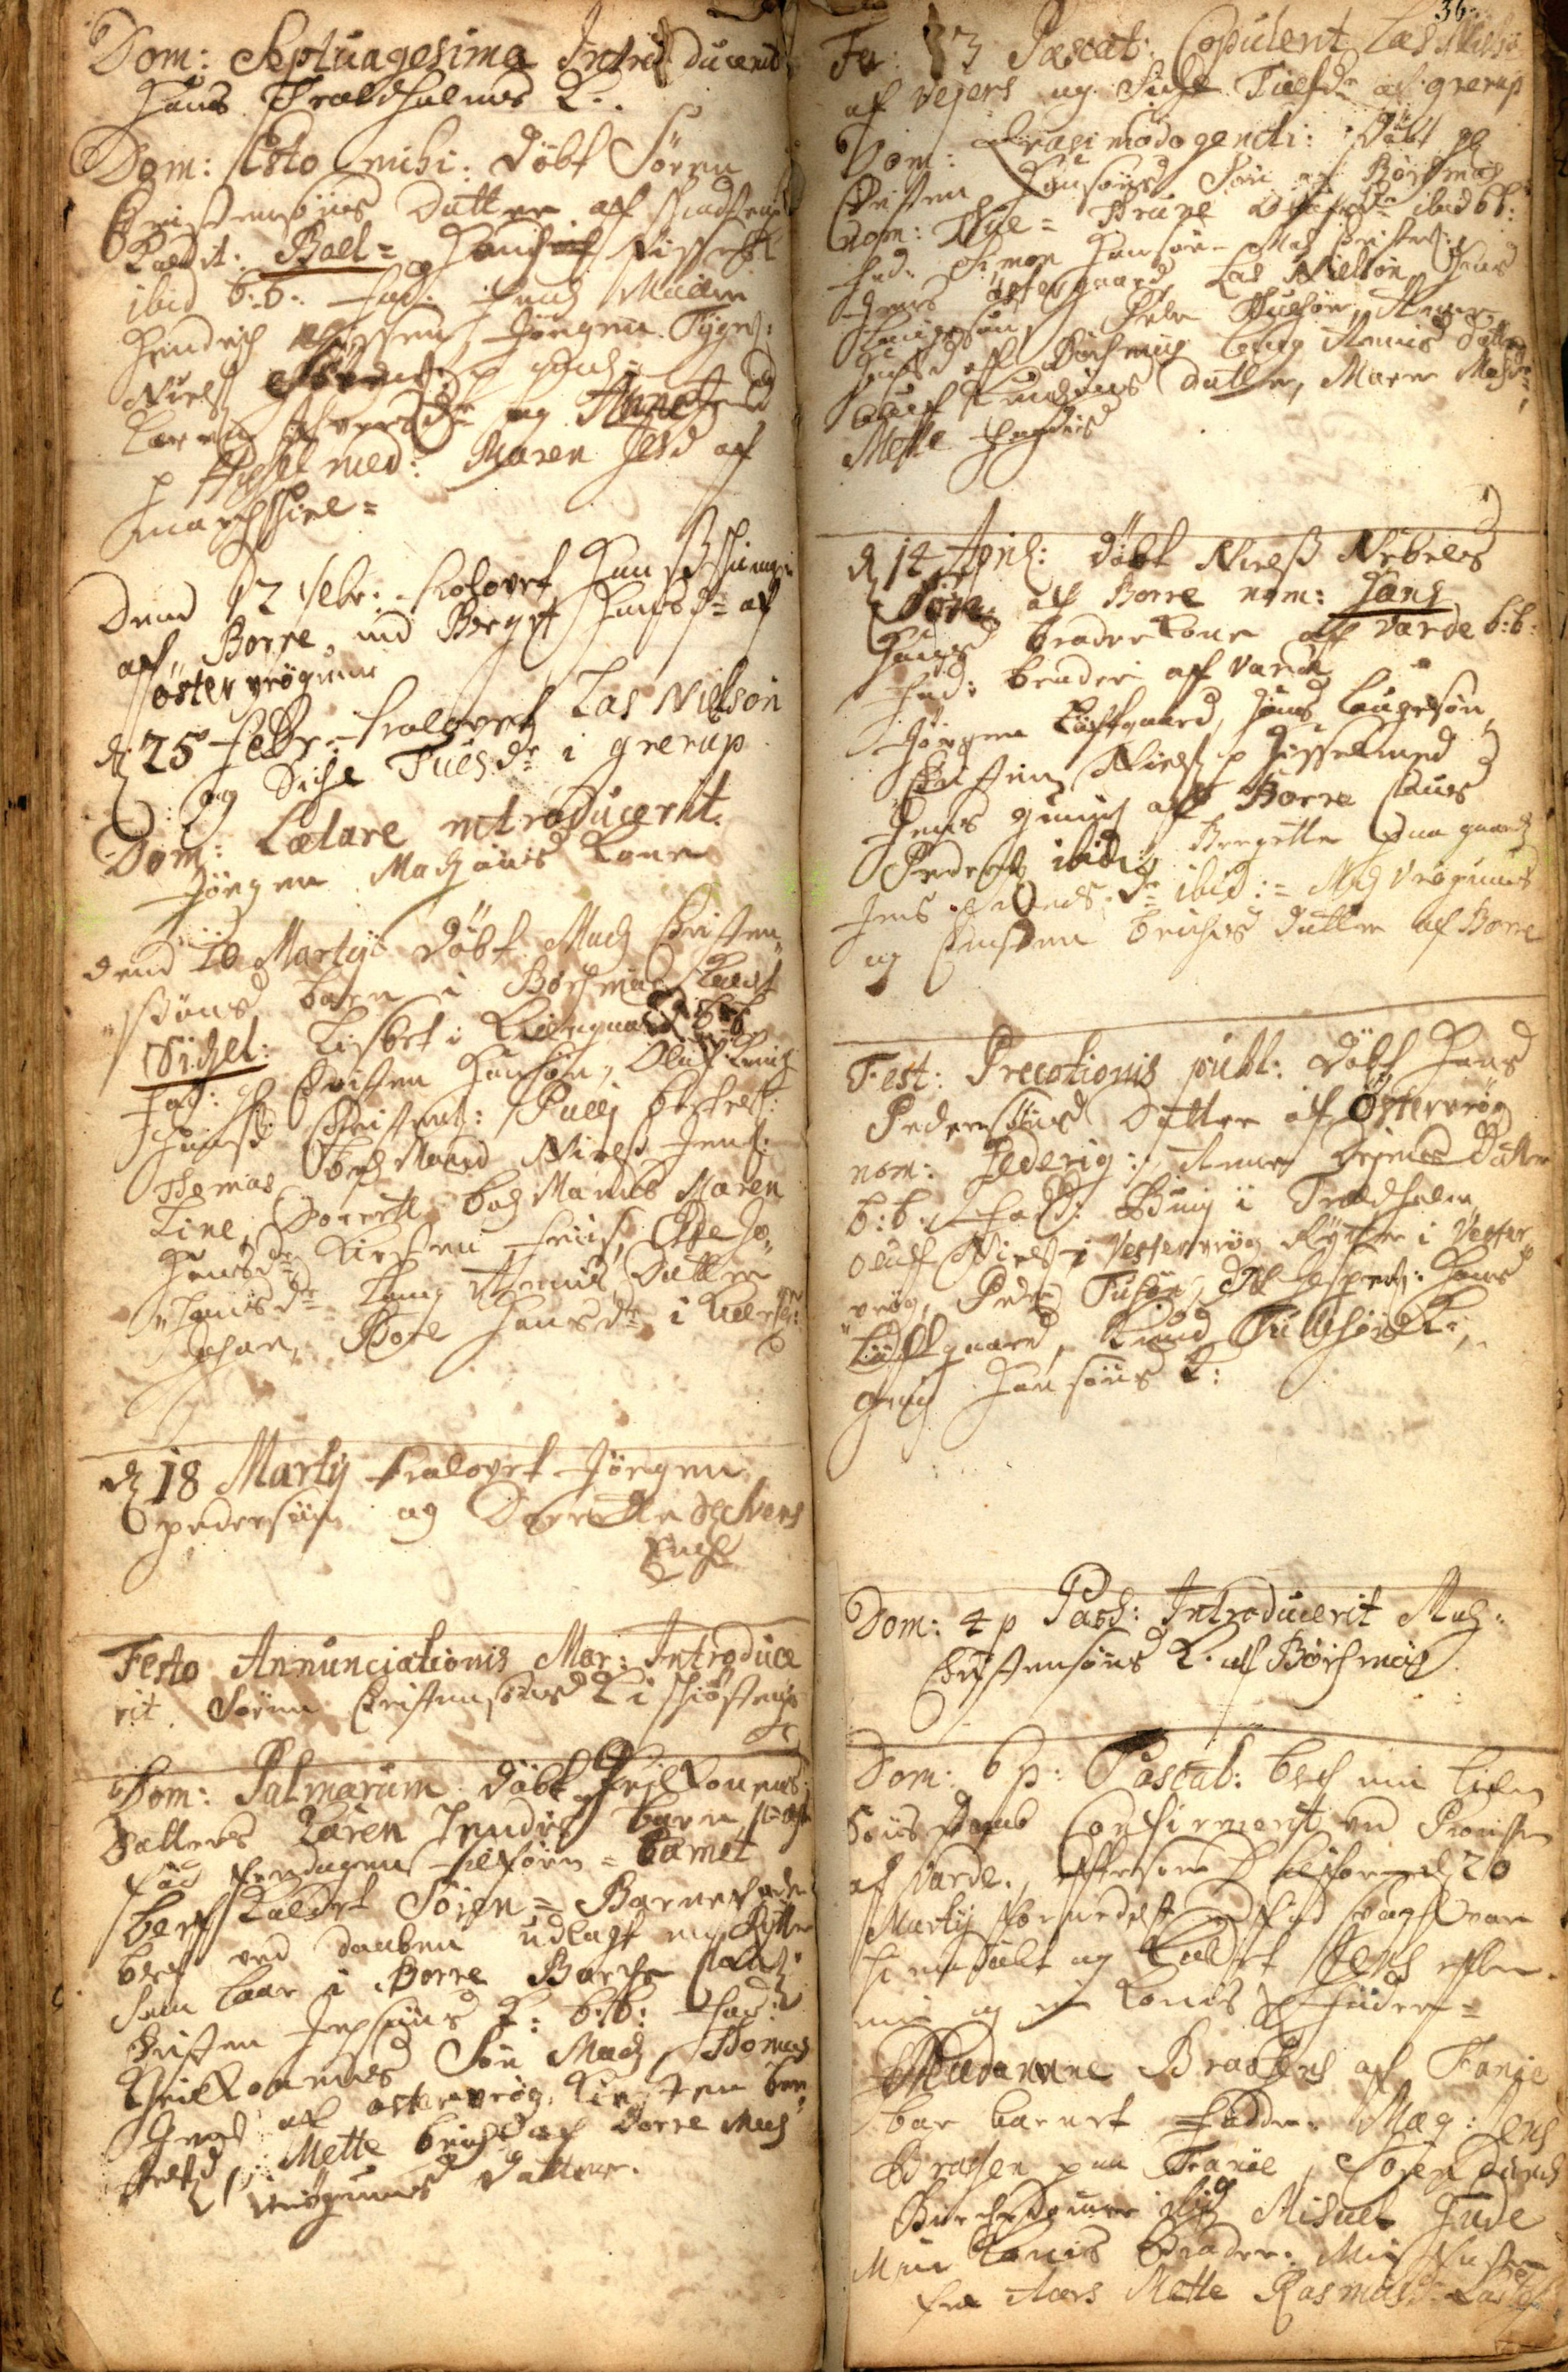DoM: Septuagesima Introducerit
Hans Troldholms K..
Dom: Esto Mihi: Døbt Søren
Christensøns Datter af Schiødstrup
kaldit: Boel= Hans ## Nisses K
ibid b:b: Fad: Frandz Møller
Hendrich Nissen, Jørgen Tyges:
Niels Sørens p ##=
Kirsten Ifversdr og Anne Jensd##
p Hisselmed: Maren Jesd af
Marchschiel=
Dend 12 Febr: trolovet Hans Schumager
af Borre med Bergit Hansdr af
Østervrøgum
D 25- Febr:- trolovet Las Nielsøn
og Siche## Tuesdr i Grerup.
Dom: Lætare introducerit
Jørgen Madzsøns Kone
Dend 10 Martij Døbt Madz Christen¬
søns Barn i Børsmøs kaldet
Sidzel: Lisbet iKiergaard b.b.
Fad: gl Christen Hansøn, Oluf Brich
Hans Christens: Pallj Bertels:
Thomas Bodz Mand Niels Jens:
Line, Dorrete Bodz Mands Maren
Hansdr Kiesten Friis, Else Jo¬
hansdr La## ##mis Datter
Johan, Boel Hansdr i Kiærgd##:
D 18 Martij trolovet Jørgen
Pedersøn og Dorrette Sl Ivers
Enche
Festo Annunciationis Mar.: Introduce
rit Søren Christensøns K i Schiødstrup
Dom: Palmarum Døbt Jejlkonens##
Datters Karen hendis Barn, ##
fød Fredagen tilforn= Barnet
blef kaldet Søren= Barnefader
blef ved Daaben udlagt en Rytter,
som laae i Borre Barchs Claus
Christen Jepsøns K: b:b: Fad:
Jeil##ouens Søn Madz, Thomas
G## af Østervrøg. Kirsten Ber¬
telsd,; Mette Brich af Borre Madz
Vrøgums Datter.
36
Fer: ## 3 Pascat: Copulerit Las Nielsøn
af Vejers og Sidzel Tuesdr af Grerup
Dom: Qvasimodogenit:i Døbt gl
Christen Hansøns Søn af Børsmos
nom: Thue= Thrine Olufsdr ibid bb:
Fad: Simon Hansøn, Madz Christens:
Jens Østergaard, Las Nielsøn, Hans
Laugesøn, Peder Thuesøn, Anne
Hansd af Børsmus ##g A##is Datter,
Oluf Knudzøns Datter, Maren Madzdr,
Mette Fogdies
D 14 April: Døbt Niels Nebels
Søn af Borre nom: Hans
Hans Broder Kone af Varde b:b:
Fad: Broder af Varde,
Jørgen Løftgaard, Hans Laugesøn,
Christen Niels p Hisselmed,
Jens Gunnis af Borre, Claus
Peders ibid: Bergitte paa gaard##
Jens Smeds## dr ibid:= Madz Vrøgums
og Christen Briches Datter af Borre
Fest: Precotionis publ: Døbt Hans
Pedersøns Datter af Østervrøg
nom: Hedevig: Anne Dejnens Datter
b:b: Fadd: Bunj i Troldholm
Oluf Niels i Vestervrøg Rytter i Vester¬
vrøg, Peder Tusøn, N Jespers: Hans
Løftgaard, Knud Tullesøn
G## Hansøns K:
Dom: 4 p Pasch: Introducerit Madz:
Christensøns K. af Børsmøs
Dom:6 p Pascat: Blef min liden
Søns Daab Confirmerit ved Prousten
af Varde. eftersom d tilforn d 20
Martij formedelst medfød Svaghed var
hiemmedøbt og kaldet Jens efter
min og min Konis Sl Fader=
Madamme Braachens af Fanøe
bar Barnet. Faddere Mag. Jens
Brachen paa Fanøe, Søren Du##
B##chedemus ibid, Michael Gude
Min Konis Broder: Min Søster
fra Aars## Mette Rasmusdr Lassøn
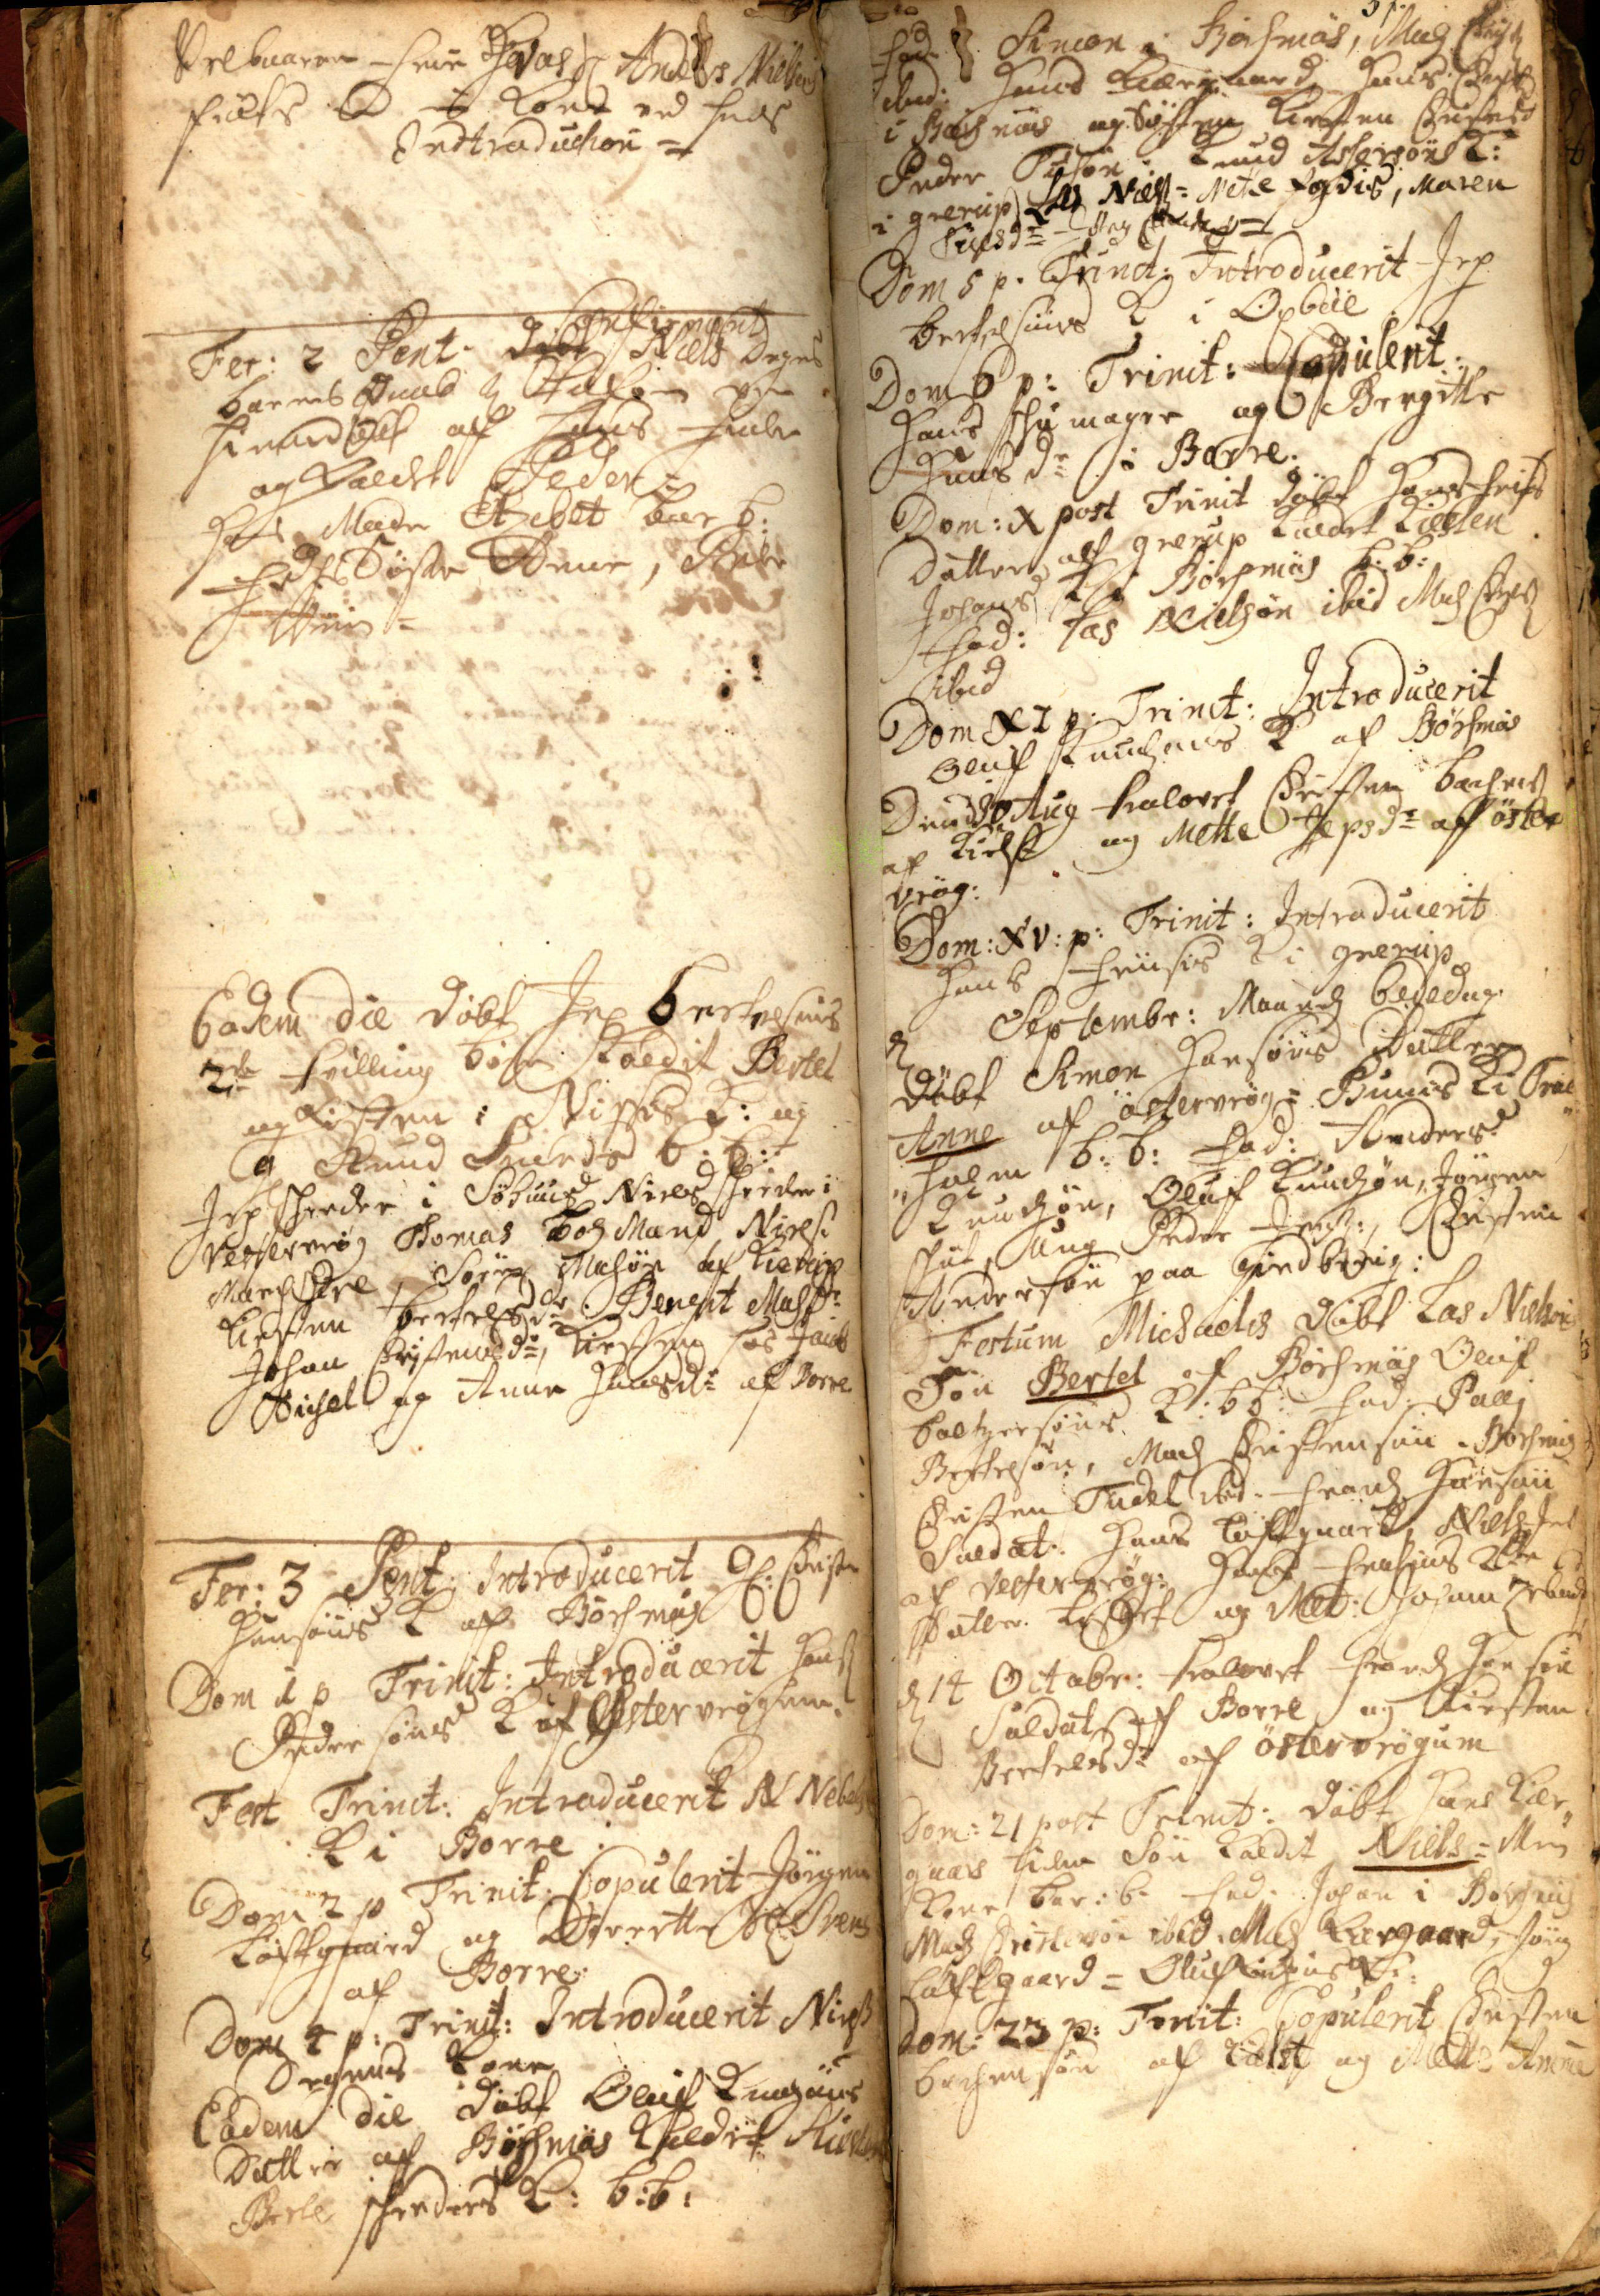Velbaarne Frue Hvas Sl Anders Nielsens
f##ts ## min Kone ved hendes
Indtroduction=
Confirmerit
Fer: 2 Pent. Niels Degns
Barns Daab ## tilforn var
hiemmedøbt af hans Fader
og kaldet Peder=
Hans M## Lisbet bar b:
Fad: hs Søster Anne, Peder
Wium=
Eodem Die Døbt Jep Bertelsøns
2de Tvilling Børn kaldit Bertel
og Kirsten; Nisses K: og
Sl Knud Smeds b:b:
Jep Schreder i Søhuus Niels Schreder i
Vestervrøg Thomas Bodz Mand, Niels
Marchschiel, Søren Madzøn af Kieru##
Kirsten Bertelsdr. Bergit Madzdr
Johanne Christensdr, Kirsten hos Jacob
Sidsel og Anne Hansdr af Borre.
Fer: 3 Pent: Introducerit Gl: Christen
Hansøns K af Børsmoøs
Dom 1 p Trinit: Introducerit Hans
Pedersøns K af Østervrøgum.
Fest Trinit: Introducerit N Nebels
K i Borre:
Dom 2 p Trinit. Copulerit Jørgen
Løftgaard og Dorrette Sl Jvers
af Borre.
Dom 4 p: Trinit: Introducerit Niels
Degens Kone
Eodem Die Døbt Oluf Knudzøns
Datter af Børsmøs kaldet Kirsten
Bertel Schreders K: b:b:
##
Fad: ## Simon i Børsmøs, Madz Christens
ibid: Hans Kiærgaard, Hans Christens
i Børsmøs og Søsteren Kirsten Christensdr
Peder Tusøn: Knud Assersøns K:
i Grerup, Las Niels= Mette Fogdis, Maren
Tuesdr Jep Christensen##=
Dom5 p.T rinit: Introducerit Jep
Bertelsøns K i Oxbøl
Dom6 p:Trinit: Copulerit
Hans Schumager og Bergitte
Hansdr i Borre.
Dom: X post Trinit døbt Hans Frisis
Datter af Grerup kaldet Kiesten
Johans K i Børsmøs b:b:
Fad: Las Nielsøn ibid Madz Christens
ibid
Dom XI p: Trinit: Introducerit
Oluf Knudzens K af Børsmøs
Dend 30 Aug Trolovet Christen Bachens
af Kielst og Mette Jepsdr af Øster
vrøg:
Dom: XV: p: Trinit: Introducerit
Hans Friisis K i Grerup
D Septembr: Maanedz Bededag
døbt Simon Hansøns Datter
Anne af Østervrøg= Bunnis K i Trol¬
holm b:b: Fad: Anders
Knudzøn, Oluf Knudzøn, Jørgen
Schøt, Ung Peder Jens: Christen
Andersøn paa Giedberig:
Festum Michaelis Døbt Las Nielsøns
Søn Bertel af Børsmøs Oluf
Baltzersøns K: bb: Fad: Pallj
Bertelsøn, Madz Christensøn i Børsmis,
Christen Tudel ibid: Frandz Hansøn
Soldat: Hans Løftgaard, Niels J##s
af Vestervrøg: Hans Frasøns## 2de
Døtre Lisbet og Met: Johanne Zebæd
D 14 Octobr: Trolovet Frandz Hansøn
Soldat af Borre og Kirsten
Bertelsdr af Østervrøgum
Dom: 21 post Trinit: Døbt Hans Kiær¬ 
gaards liden Søn kaldit Niels= Min
Kone Bar: b. Fad. Johan i Børsmøs
Madz Christensøn ibid: Madz Kiærgaard, Jørg
Løftgaard= Oluf Knudzøns K:
Dom: 23 p:Trinit: Copilerit Christen
Bachebsøn af Kielst og Mette An##